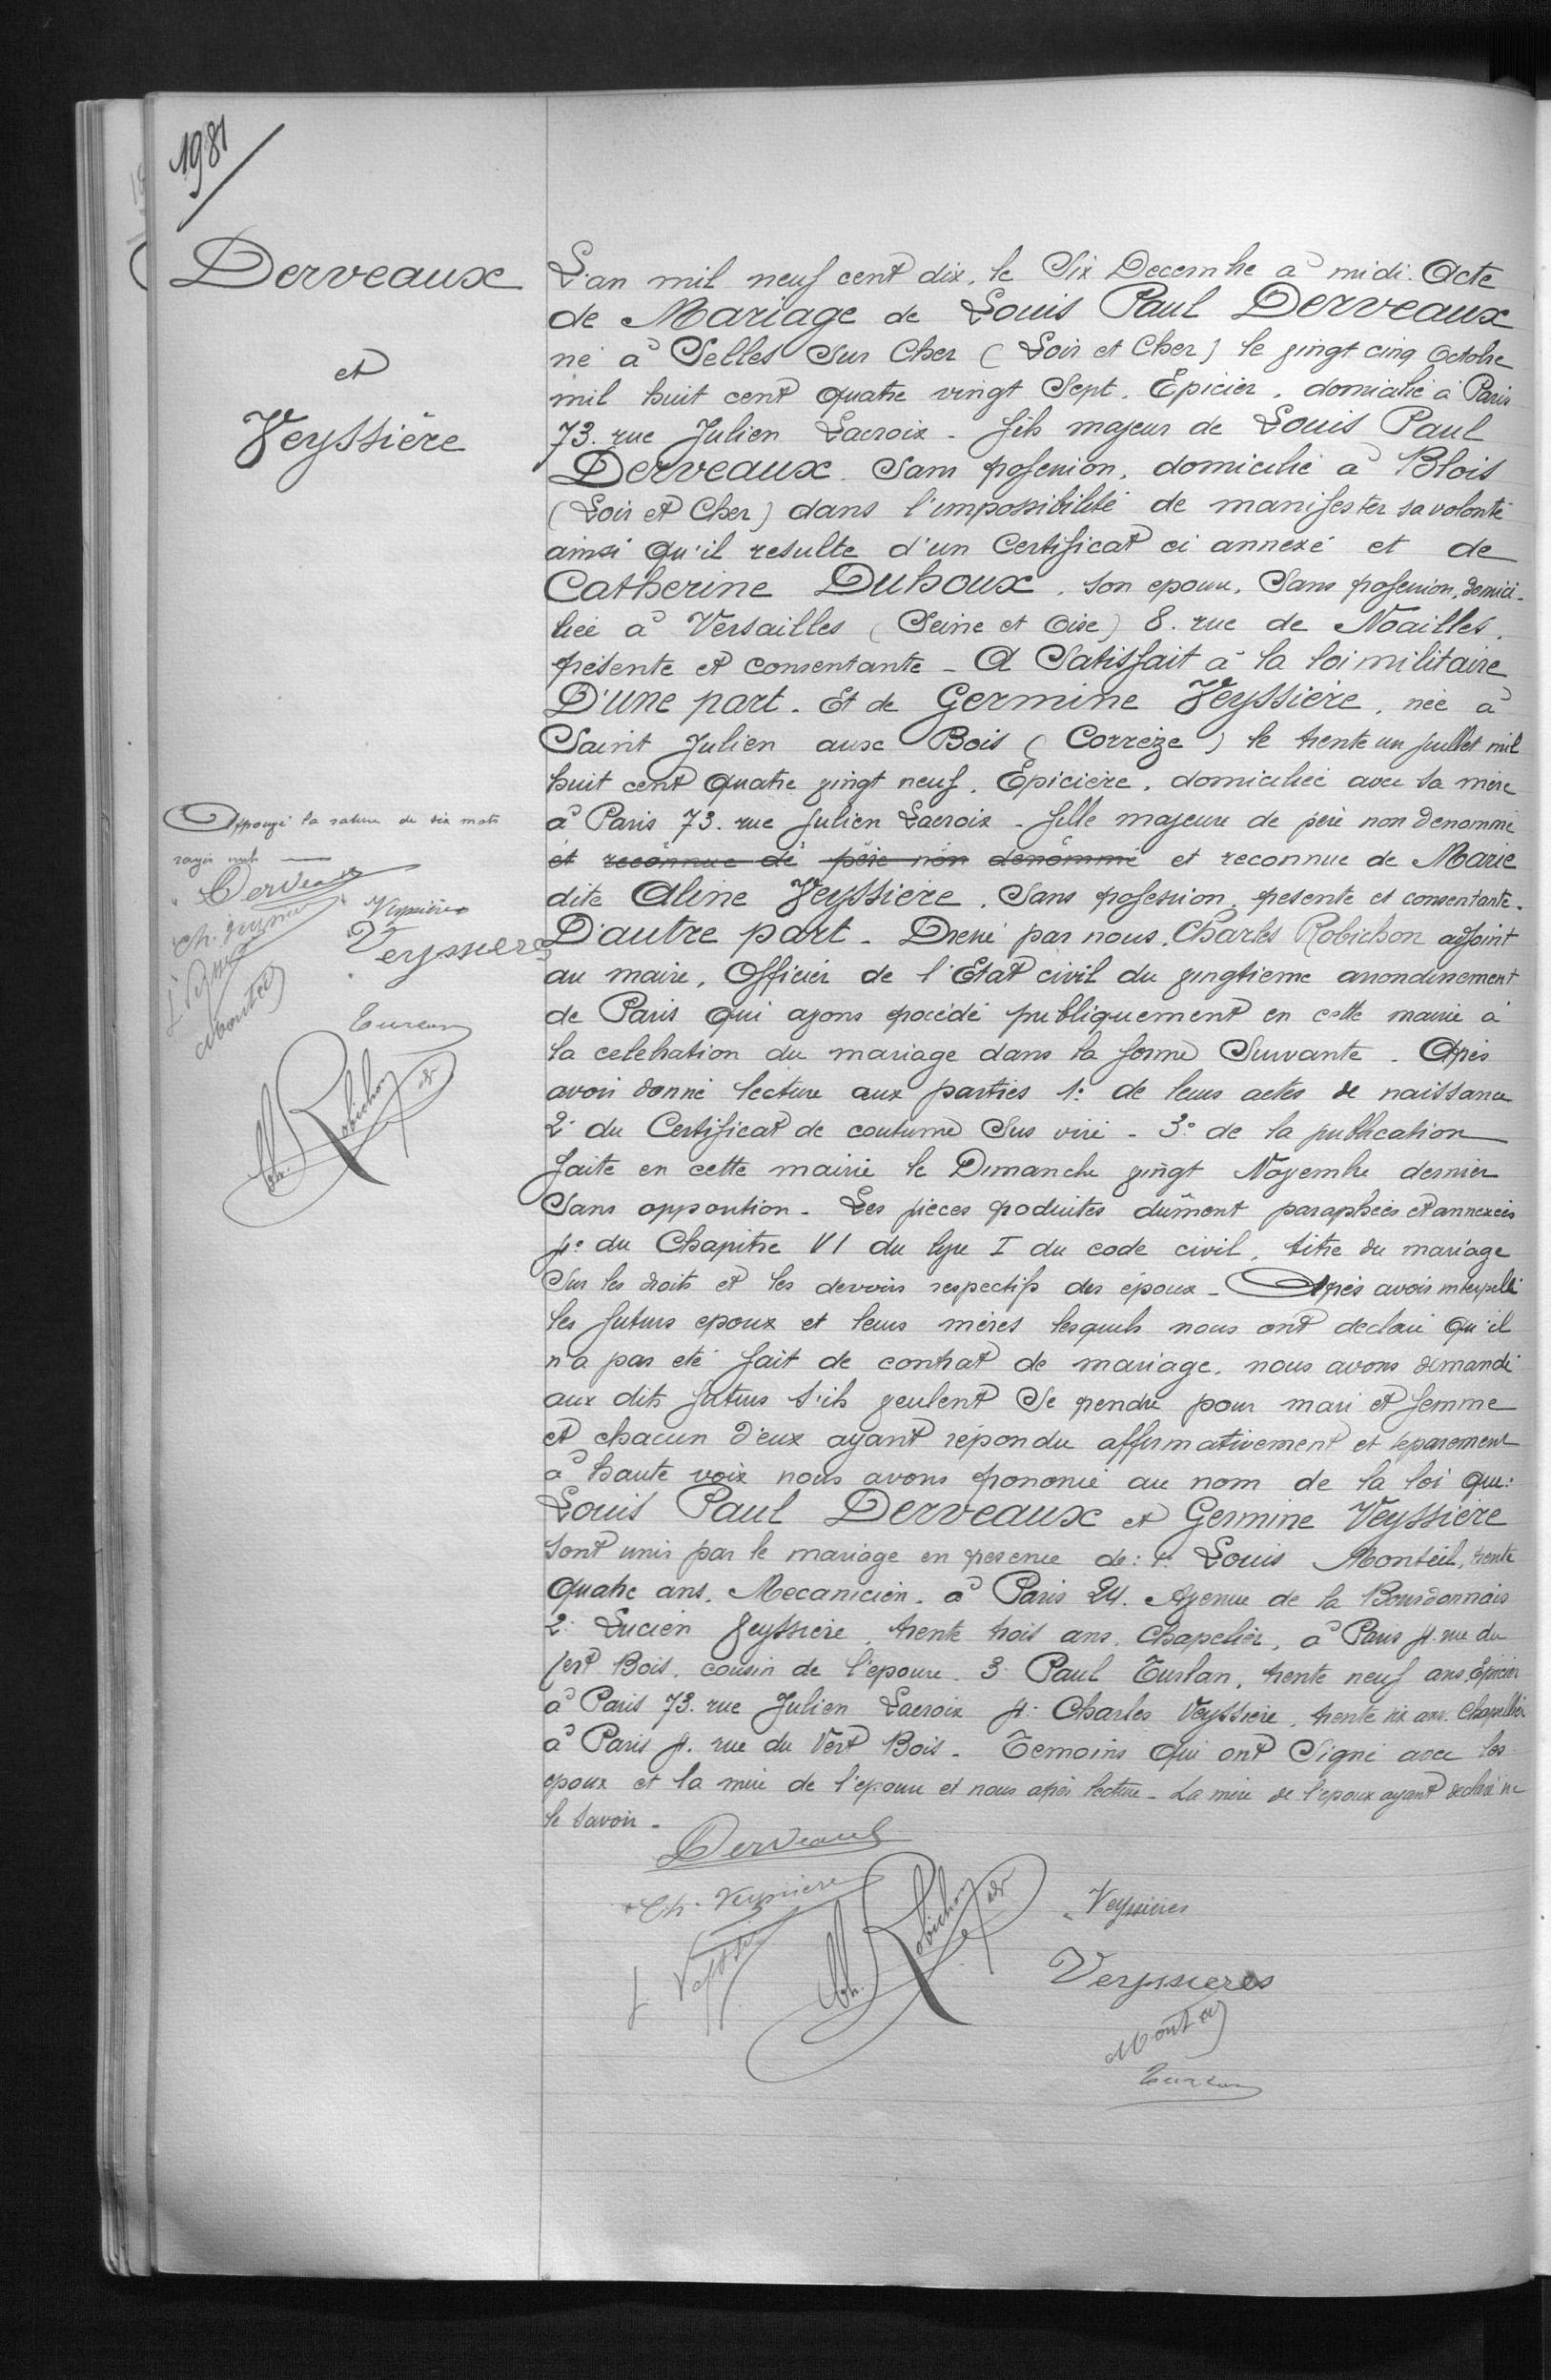1981
Derveaux
et
Veyssière
L'an mil neuf cent dix, le Six Decembre à midi. Acte
de Mariage de Louis Paul Derveaux
né à Selle sur Cher (Loir et Cher) le vingt cinq Octobre
mil huit cent quatre vingt Sept. Epicier domicilié à Paris
73 rue Julin Lacroix fils majeur de Louis Paul
Derveaux sans profession, domicilié à Blois
(Loir et Cher) dans l'impossibilité de manifester sa volonté
ainsi qu'il resulte d'un Certificat ci annexé et de
Catherine Duboux, son epoux, Sans profession domici-
liée a Versailles (Seine et Oise) 8 rue de Noailles
présente et consentante-A Satisfait à la loi militaire
D'une part. Et de Germine Veyssière, née à
Saint Julien aux Bois (Corrèze) le trente un juillet mil
huit cent quatre vingt neuf. Epicière domiciliée avec sa mère
à Paris, 73 rue Julien Lacroix -fille majeure de père non denommé
et reconnue de père non dénommé et reconnue de Marie
dite Aline Veyssière. Sans profession. présente et consentante.
D'autre part -Dressé par nous. Charles Robichon adjoint
au maire, Officier de l'Etat civil du vingtième arrondissement
de Paris qui ayons procédé publiquement en cette mairie à
la celebration du mariage dans la forme Suivante. Après
avoir donné lecture aux parties 1° de leurs actes de naissance
2° du Certificat de coutume sus viri-3° de la publication
faite en cette mairie le Dimanche vingt Noyembre dernier
Sans opposition. Ses pièces produites dûment paraphées et annexées
4° du Chapitre VI du livre I du code civil, titre du mariage
Sur les droits et les devoirs respectifs des époux. Après avoir interpellé
les futurs epoux et leurs mères lesquels nous ont déclaré qu'il
n'a pas été fait de contrat de mariage. nous avons demandé
aux dits futurs s'ils veulent se prendre pour mari et femme
et chacun d'eux ayant répondu affirmativement et séparement
à haute voix nous avons prononcé au nom de la loi que
Louis Paul Derveaux et Germine Veyssière
sont unis par le mariage en presence de: Louis Monteil, trente
quatre ans Mecanicien. à Paris 24 Avenue de la Bourdonnais
2 Lucien Veyssière trente trois ans. Chapelier, à Paris, 4 rue du
Vert Bois. cousin de l'épouse 3. Paul Tourlan, trente neuf ans Epicier
à Paris 73 rue Julien Lacroix 4 Charles Veyssière. trente six ans Chapelier
à Paris 4. rue du Vert Bois. Temoins qui ont Signé avec les
epoux et la mère de l'epouse et nous après lecutre. La mère de l'époux ayant declaré ne
le savoir.
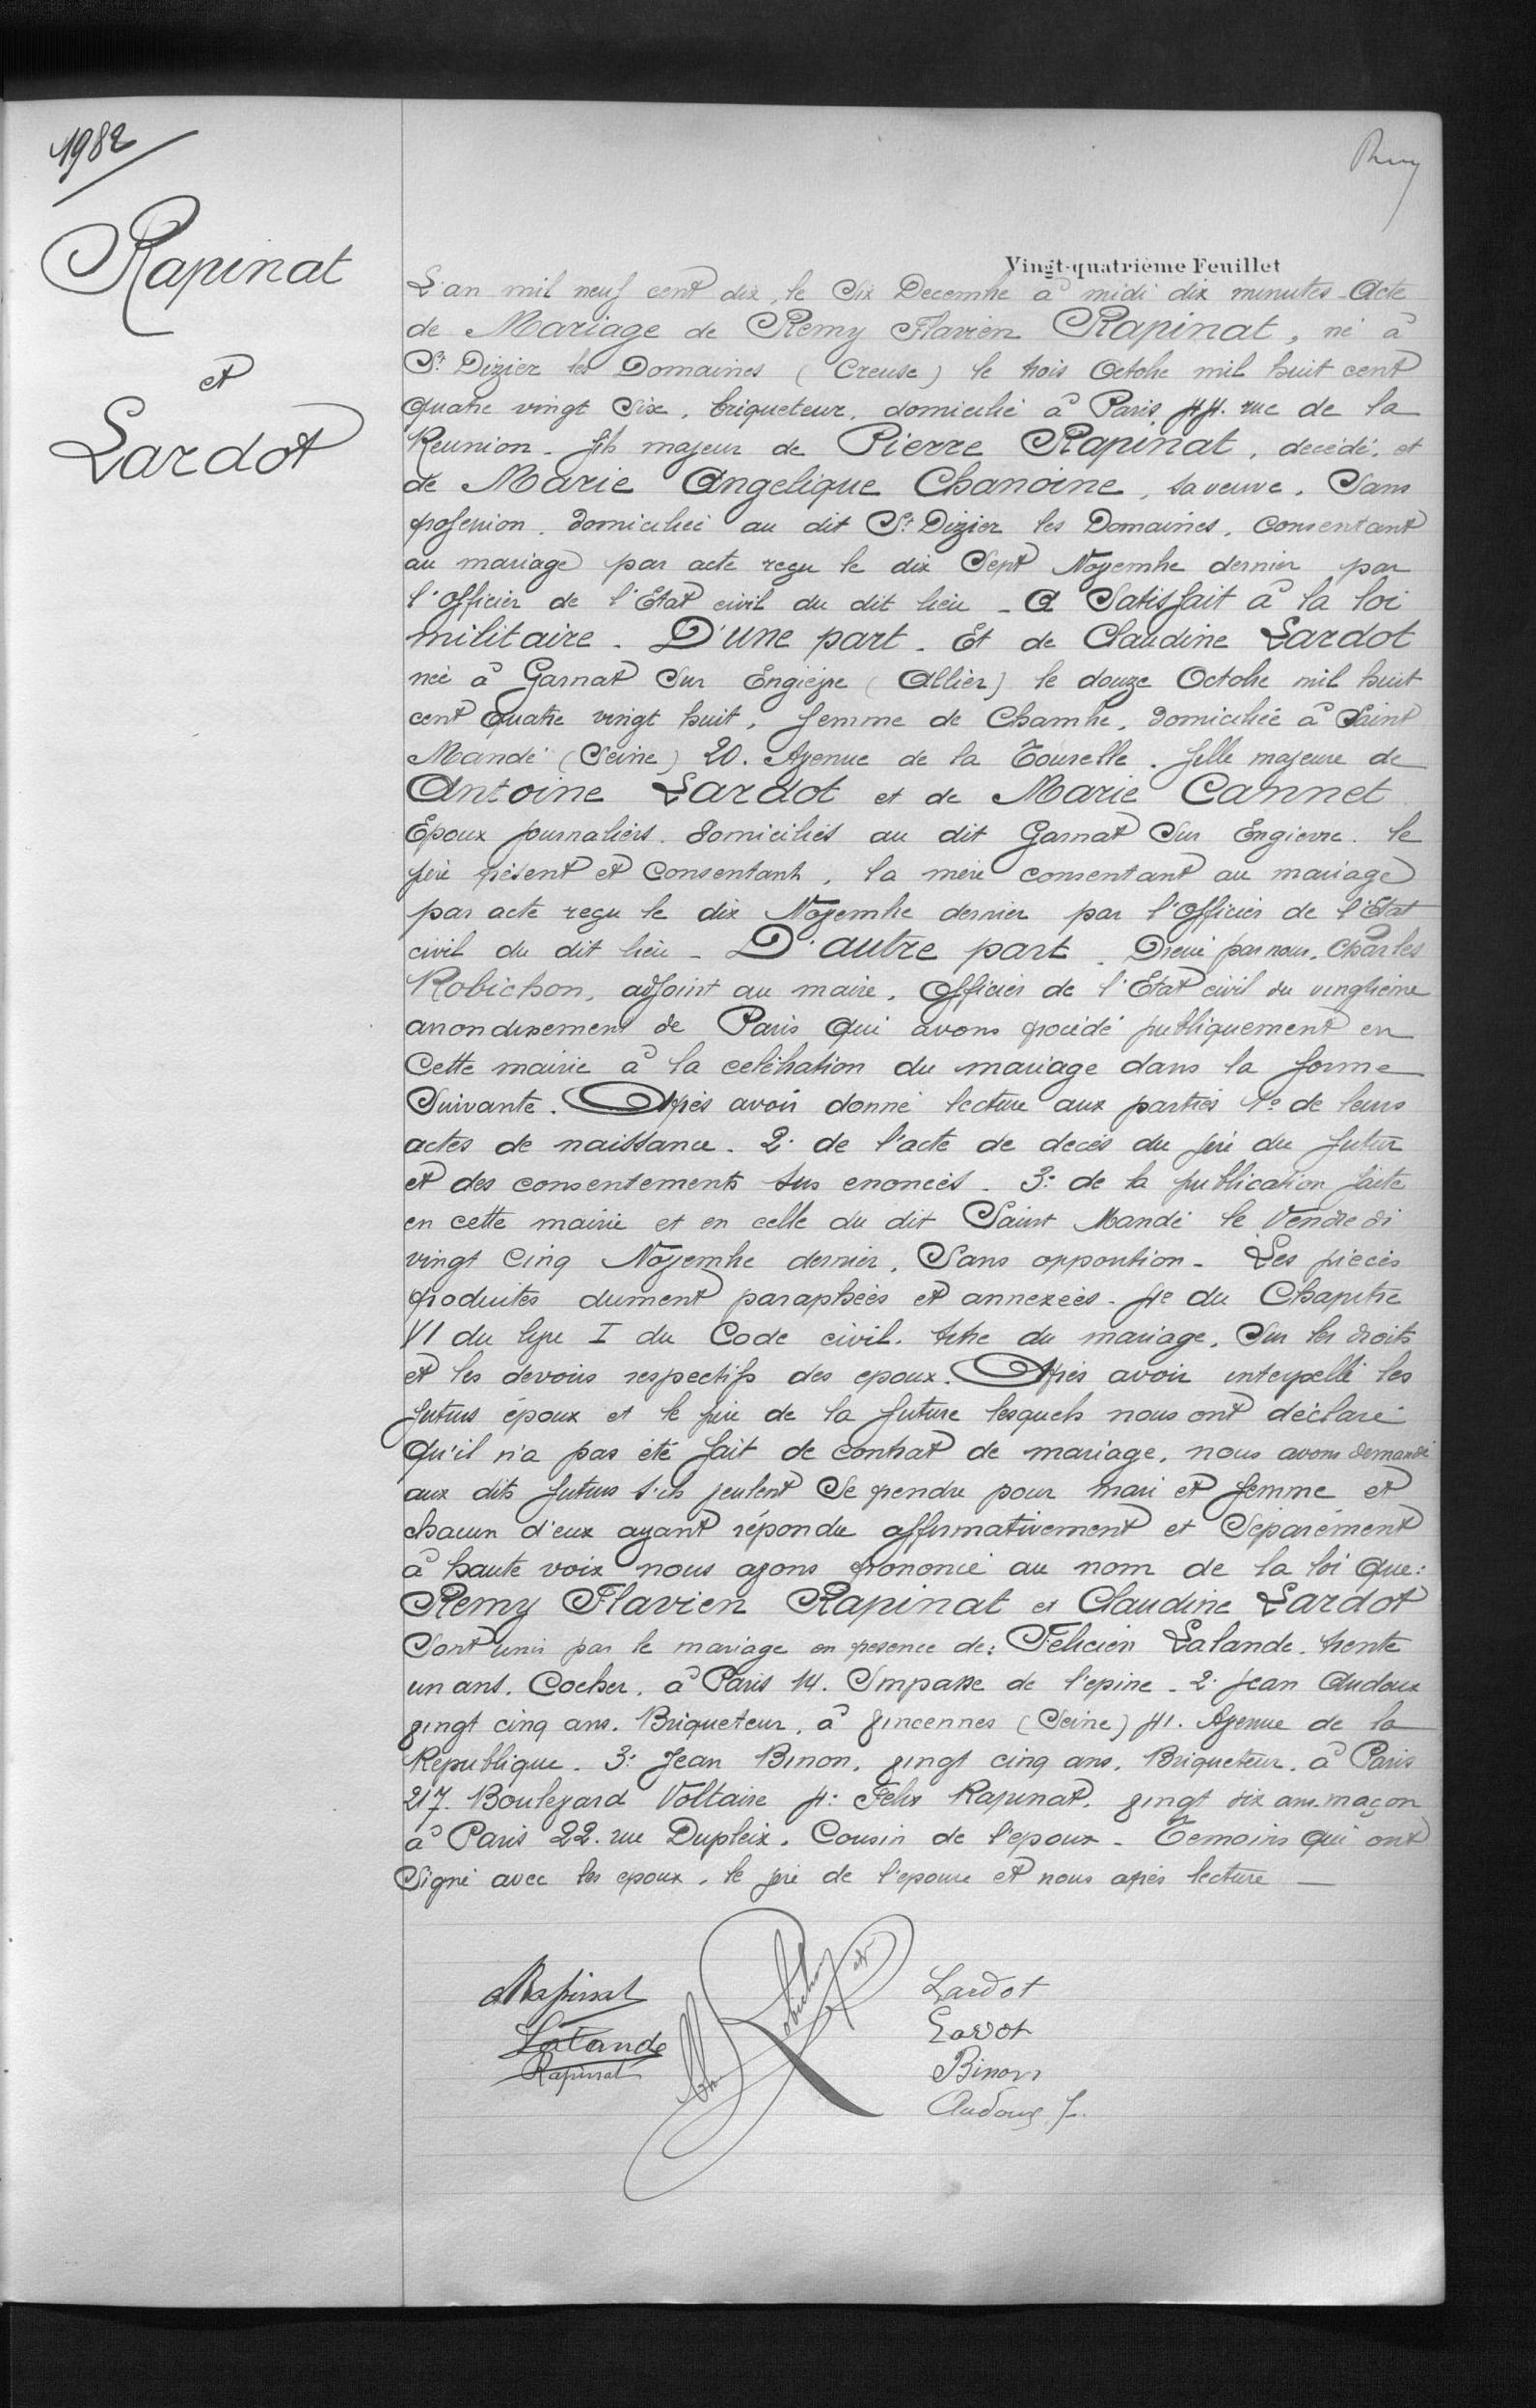1982
Rapinat
et
Lardot
L'an mil neuf cent dix, le dix Decembre à midi dix minutes Acte
de Mariage de Remy Flavien Rapinat, né à
St Dizier les Domaines (Creuse) le trois Octobre mil huit cent
quatre vingt six, briqueteur domicilié à Paris 44 rue de la
Reunion, fils majeur de Pierre Rapinat, décédé, et
de Marie Angelique Chanoine, sa veuve, Sans
profession, domiciliée au dit St Dizier les Domaines, Consentant
au mariage par acte reçu le dix Sept Novembre dernier par
l'Officier de l'Etat civil du dit lieu -A Satisfait à la loi
militaire. D'une part. Et de Claudine Sardot
née à Garnat sur Engièvre (Allier) le douze Octobre mil huit
cent quatre vingt huit, femme de Chambre, domiciliée à Saint
Mandé (Seine) 20. Avenue de la Tourelle, fille majeure de
Antoine Sardot et de Marie Cannet
Epoux journaliers domiciliés au dit Garnat sur Engièvre. le
père présent et consentant. la mère consentant au mariage
par acte reçu le dix Novembre dernier par l'Officier de l'Etat
civil du dit lieu-D'autre part. Dressé par nous. Charles
Robichon, adjoint au maire. Officier de l'Etat Civil du vingtieme
arrondissement de Paris qui avons procédé publiquement en
cette mairie à la celebration du mariage dans la forme
suivante. Après avoir donné lecture aux parties 1° de leurs
actes de naissance. 2° de l'acte de decès du père du futur
et des consentement sus enoncées. 3° de la publication faite
en cette mairie et en celle du dit Saint Mandé le Vendredi
vingt cinq Novembre dernier. Sans opposition -Les pièces
produites dument paraphées et annexées. 4° du Chapitre
VI du livre I du Code civil. titre du mariage. Sur les droits
et les devoirs respectifs des epoux. Après avoir interpellé les
futurs époux et le père de la future lesquels nous ont déclaré
qu'il n'a pas été fait de contrat de mariage. nous avons demandé
aux dits futurs s'il veulent se prendre pour mari et femme. et
chacun d'eux ayant répondu affirmativement et séparément
à haute voix nous ayons prononcé au nom de la loi que:
Remy flavien Rapinat et Claudine Lardot
Sont unis par le mariage en presence de: Felicien Lalande. trente
un ans. Cocher à Paris 14. Impasse de l'epine -2° Jean Andoue
vingt cinq ans. Briqueteur à Vincennes (Seine) 41. Avenue de la
Republique. 3° Jean Binon. vingt cinq ans. Briqueteur à Paris
217. Boulevard Voltaire. 4: Felix Rapunat vingt six ans. maçon
à Paris 22. rue Dupleix. Cousin de l'epoux. Temoins qui ont
Signé avec les epoux. le père de l'epouse et nous après lecture -